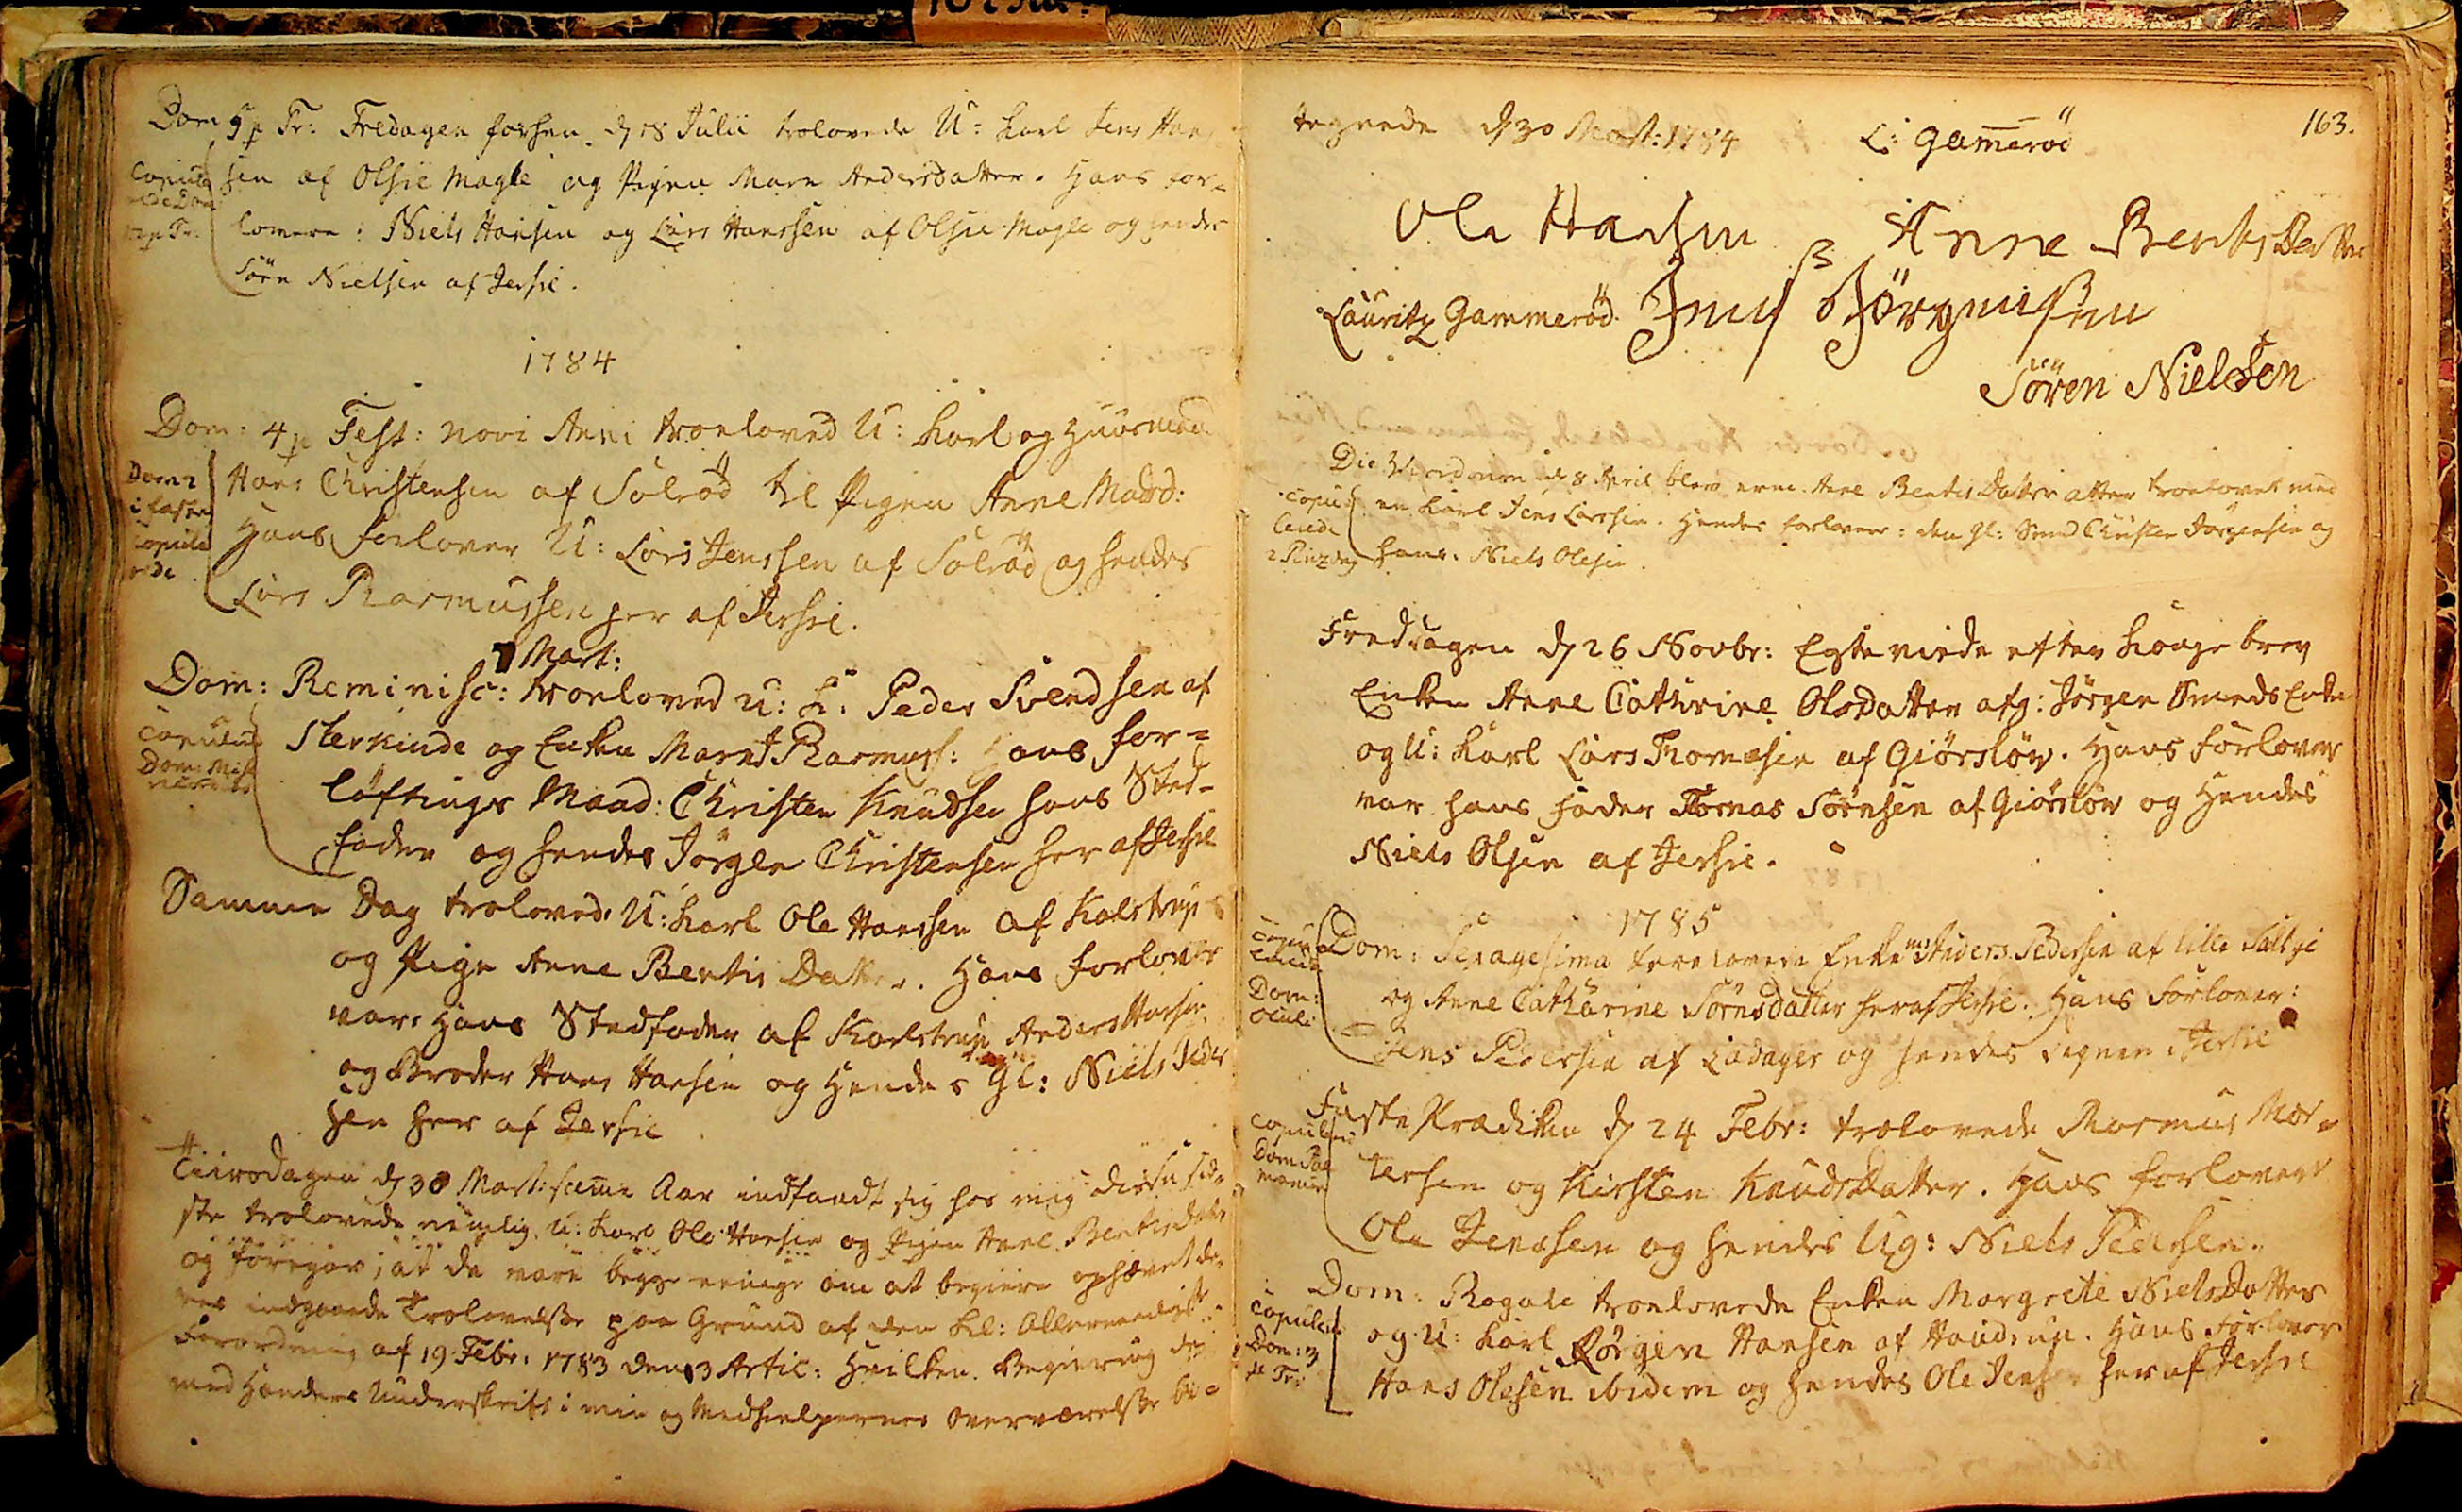Dom: 5 p Tr: Fredagen forhen d 18 Julii trolovede U: karl Jens Hans
sen af Olsie Magle og Pigen Marn Andersdatter. Hans for¬
lovere: Niels Hansen og Lars Hanssen af Olsie Magle og hendes
Sørn Nielsen af Jersie.
Copule
rede Dom:
12 p Tr:
1784
Dom: 4 p Fest: novi Anni troeloved U: karl og Huusmand
Hans Christensen af Solrød til Pigen Anne Madsd:
Hans forlover U: Lars Jenssen af Solrød og hendes
Lars Rasmussen her af Jersie
Dom 2
i Faste
Copule
rede
7 Mart:
Dom: Reminisc: troeloved u: K: Peder Svendsen af
Sterkinde og Enke Marn J Rasmuss: Hans for¬
løftings Mand: Christen Knudsen hans Sted¬
fader og hendes Jørgen Christensen her af Jersie
Copulered
Dom: Mise
ricordias
Samme Dag trolovede U:karl Ole Hanssen af Kalstrup
og Pige Anne Bentis Datter. Hans forlover
var Hans Stedfader af Karlstrup Anders Hansen
og Broder Hans Hansen og Hendes Gl: Niels Peder
sen her af Jersie.
Tiirsdagen d 30 Mart: samme Aar indfandt sig hos mig disse sid¬
ste trolovede nemlig u: karl Ole Hansen og Pigen Anne Bentisdatter
og foregav; at de vare begge eenige om at begiere ophævet de¬
res indgaaede Trolovelse paa Grund af den Kl: Allernaadigst
Forordning af 19 Febr. 1783 den 3 Artic: Hvilken Begiering de
med Hænders Underskrift i min og Medhielpernes Overværelse be¬
163.
tegnede d 30 Mart: 1784
L: Gammerød
Ole Hansen
Anne Bentis Datter
Lauritz Gammerød
Jens Jørgensen 
Søren Nielsen
Die Viridium d 8 April blev erm##. Anne Bentis Datter atter troeloved med
en karl Jens Larssen. Hendes forlover: den Gl: Smed Christen Jørgensen og
hans. Niels Olesen.
Copu¬ 
lerede
2 Pinzdag
Freddagen d 26 Novbr: Egteviede efter Kongebrev
Enken Anne Cathrine Olsdatter afg: Jørgen Smeds Enke
og U: karl Lars Thomæsen af Giørsløv. Hans Forlover
var hans Fader Thomas Sørnsen af Giørsløv og Hendes
Niels Olsen af Jersie.
1785.
Dom: Sexagesima troelovede Enke md Anders Pedersen af lille Salbye
og Anne Catharine Sørnsdatter heraf Jersie. Hans Forlover:
Jens Pedersen af Ladager og hendes Degnen i Jersie
Copu¬
lerede
Dom:
Oculi
Faste Prædiken d 24 Febr: trolovede Rasmus Mor¬
tensen og Kirsten KnudsDatter. Hans forlovere
Ole Jenssen og hendes Ug: Niels Pedersen.
Copulered
Dom Pal
marum
Dom: Rogate troelovede Enken Margrete NielsDatter
og U: karl Jørgen Hansen af Haudrup. Hans Forlover
Hans Olesen ibidem og hendes Ole Jensen her af Jersie
Copulered
Dom: 3
p Tr: